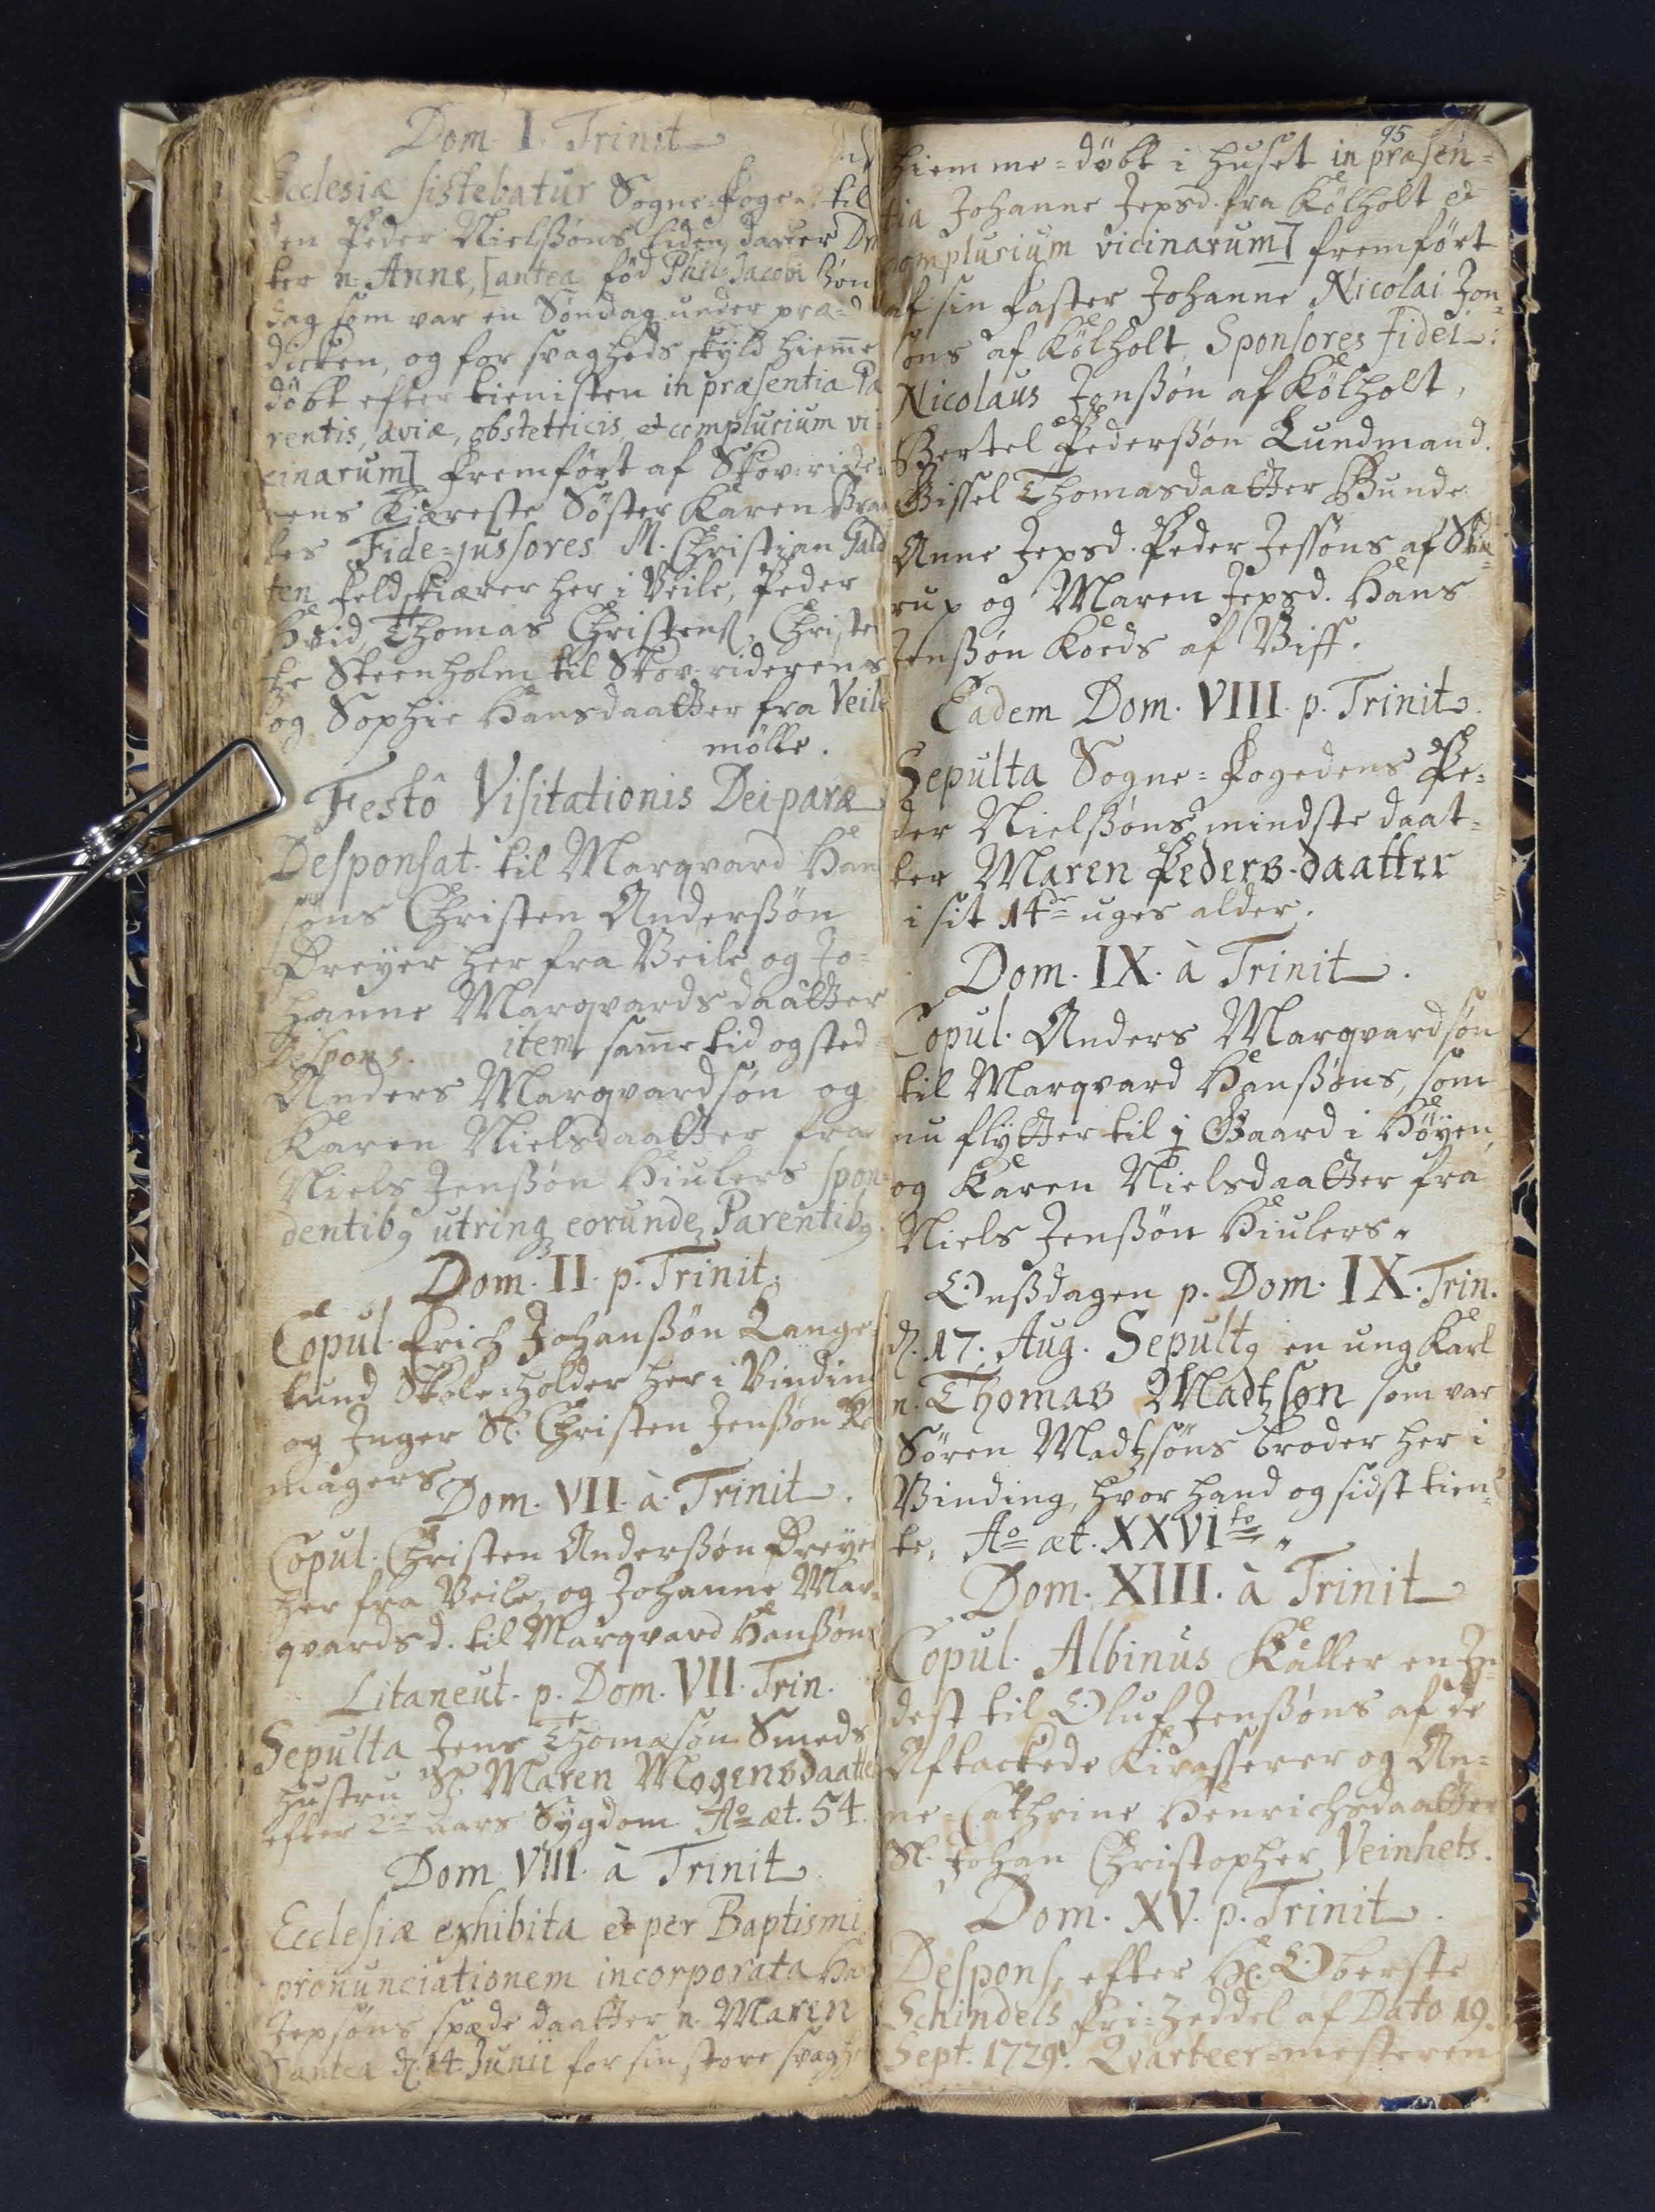Dom. I. Trinit
Ecclesiæ sistebatur Sogne=Foge 
en Peder Nielssøns liden daa 
ter n. Anna, [antea fød Philip Jacobi
dag som var en Søndag under præ¬
dicken, og for svagheds skyld hiemme
døbt efter tienisten in præsentia Pa
rentis, aviæ, obstetricis et complurium vi¬
cinarum] fremført af Skov=ride¬
ens Kiæreste Søster Karen Braa 
tes Fide=jussores M. Christian Gald
ten Feldskiærer her i Veile, Peder
Hvid, Thomas Christens, Christe 
ze Steenholm til Skov=riderns
og Sophie Hansdaatter fra Veile
Mølle.
Festo Visitationis Dei-paræ
Desponsat til Marqvard Han
søns Christen Anderssøn
Dreyer her fra Veile og Jo¬
hanne Marqvardsdaatter
Despons.
item samme tid og sted
Anders Marqvardsøn og
Karen Nielsdaatter fra
Niels Jenssøn Hiulers Spon¬
dentibq utringz eorundez Parentibq
Dom. II. p. Trinit
Copul. Erich Johanssøn Lange¬
lund Skole=holder her i Vindin 
og Inger Sl. Christen Jenssøn Rø 
magers.
Dom. VII. a. Trinit
Copul. Christen Anderssøn Dreye 
her fra Veile, og Johanne Mar¬
qvardsd. til Marqvard Hanssøns
Litaneut. p. Dom. VII. Trin
Sepulta Jens Thomæsøn Smeds 
hustru Sl. Maren Mogensdaatter
efter 2de Aars Sygdom Ao æt. 54.
Dom. VIII. a Trinit
Ecclesiæ exhibita et per Baptismi 
pronunciationem incorporata Ha 
Jepsøns spæde daatter n. Maren 
[ antea d. 14 Junii for sin store svaghe 
95
hiemme = døbt i huset in præsen¬
tia Johanne Jepsd. fra Kølholt et 
complurium vicinarum] fremført
af sin Faster Johanne Nicolai Jon¬
søns af Kølholt Sponsores fidei
Nicolaus Jonssøn af Kølholt
Bertel Pederssøn Lundmand.
Gissel Thomasdaatter Bunde
Anne Jepsd. Peder Jessøns af
rup og Maren Jepsd. Hans
Jenssøn Koeds af Viff
Eadem Dom. VIII. p. Trinit
Sepulta Sogne=Fogedens Pe¬
der Nielssøns mindste daat¬
ter Maren Peders-daatter
i sit 14de uges alder.
Dom. IX. a Trinit.
Copul. Anders Marqvardsøn
til Margvard Hanssøns, som
nu flytter til 1 Gaard i Høyen
og Karen Nielsdaatter fra
Niels Jenssøn Hiulers.
Onssdagen p. Dom. IX .Trin.
d. 17. Aug. Sepultq en ung Karl
n. Thomas Madtzsøn som var
Søren Madtzsøns broder her i
Vinding, hvor hand og sidst tien¬
te, Ao æt. XXVIto
Dom. XIII. a Trinit
Copul. Albinus Kaller en In¬
dest til Oluf Jenssøns af de
Aftackede Kirasserer og An¬
ne = Cathrine Hentichsdaatter
Sl. Johan Christropher Veinhets.
Dom. XV. p. Trinit
Despons efter Hl Oberste
Schindels fri=Zeddel af Dato 19.
Sept. 1729 Qvarteer=mesteren
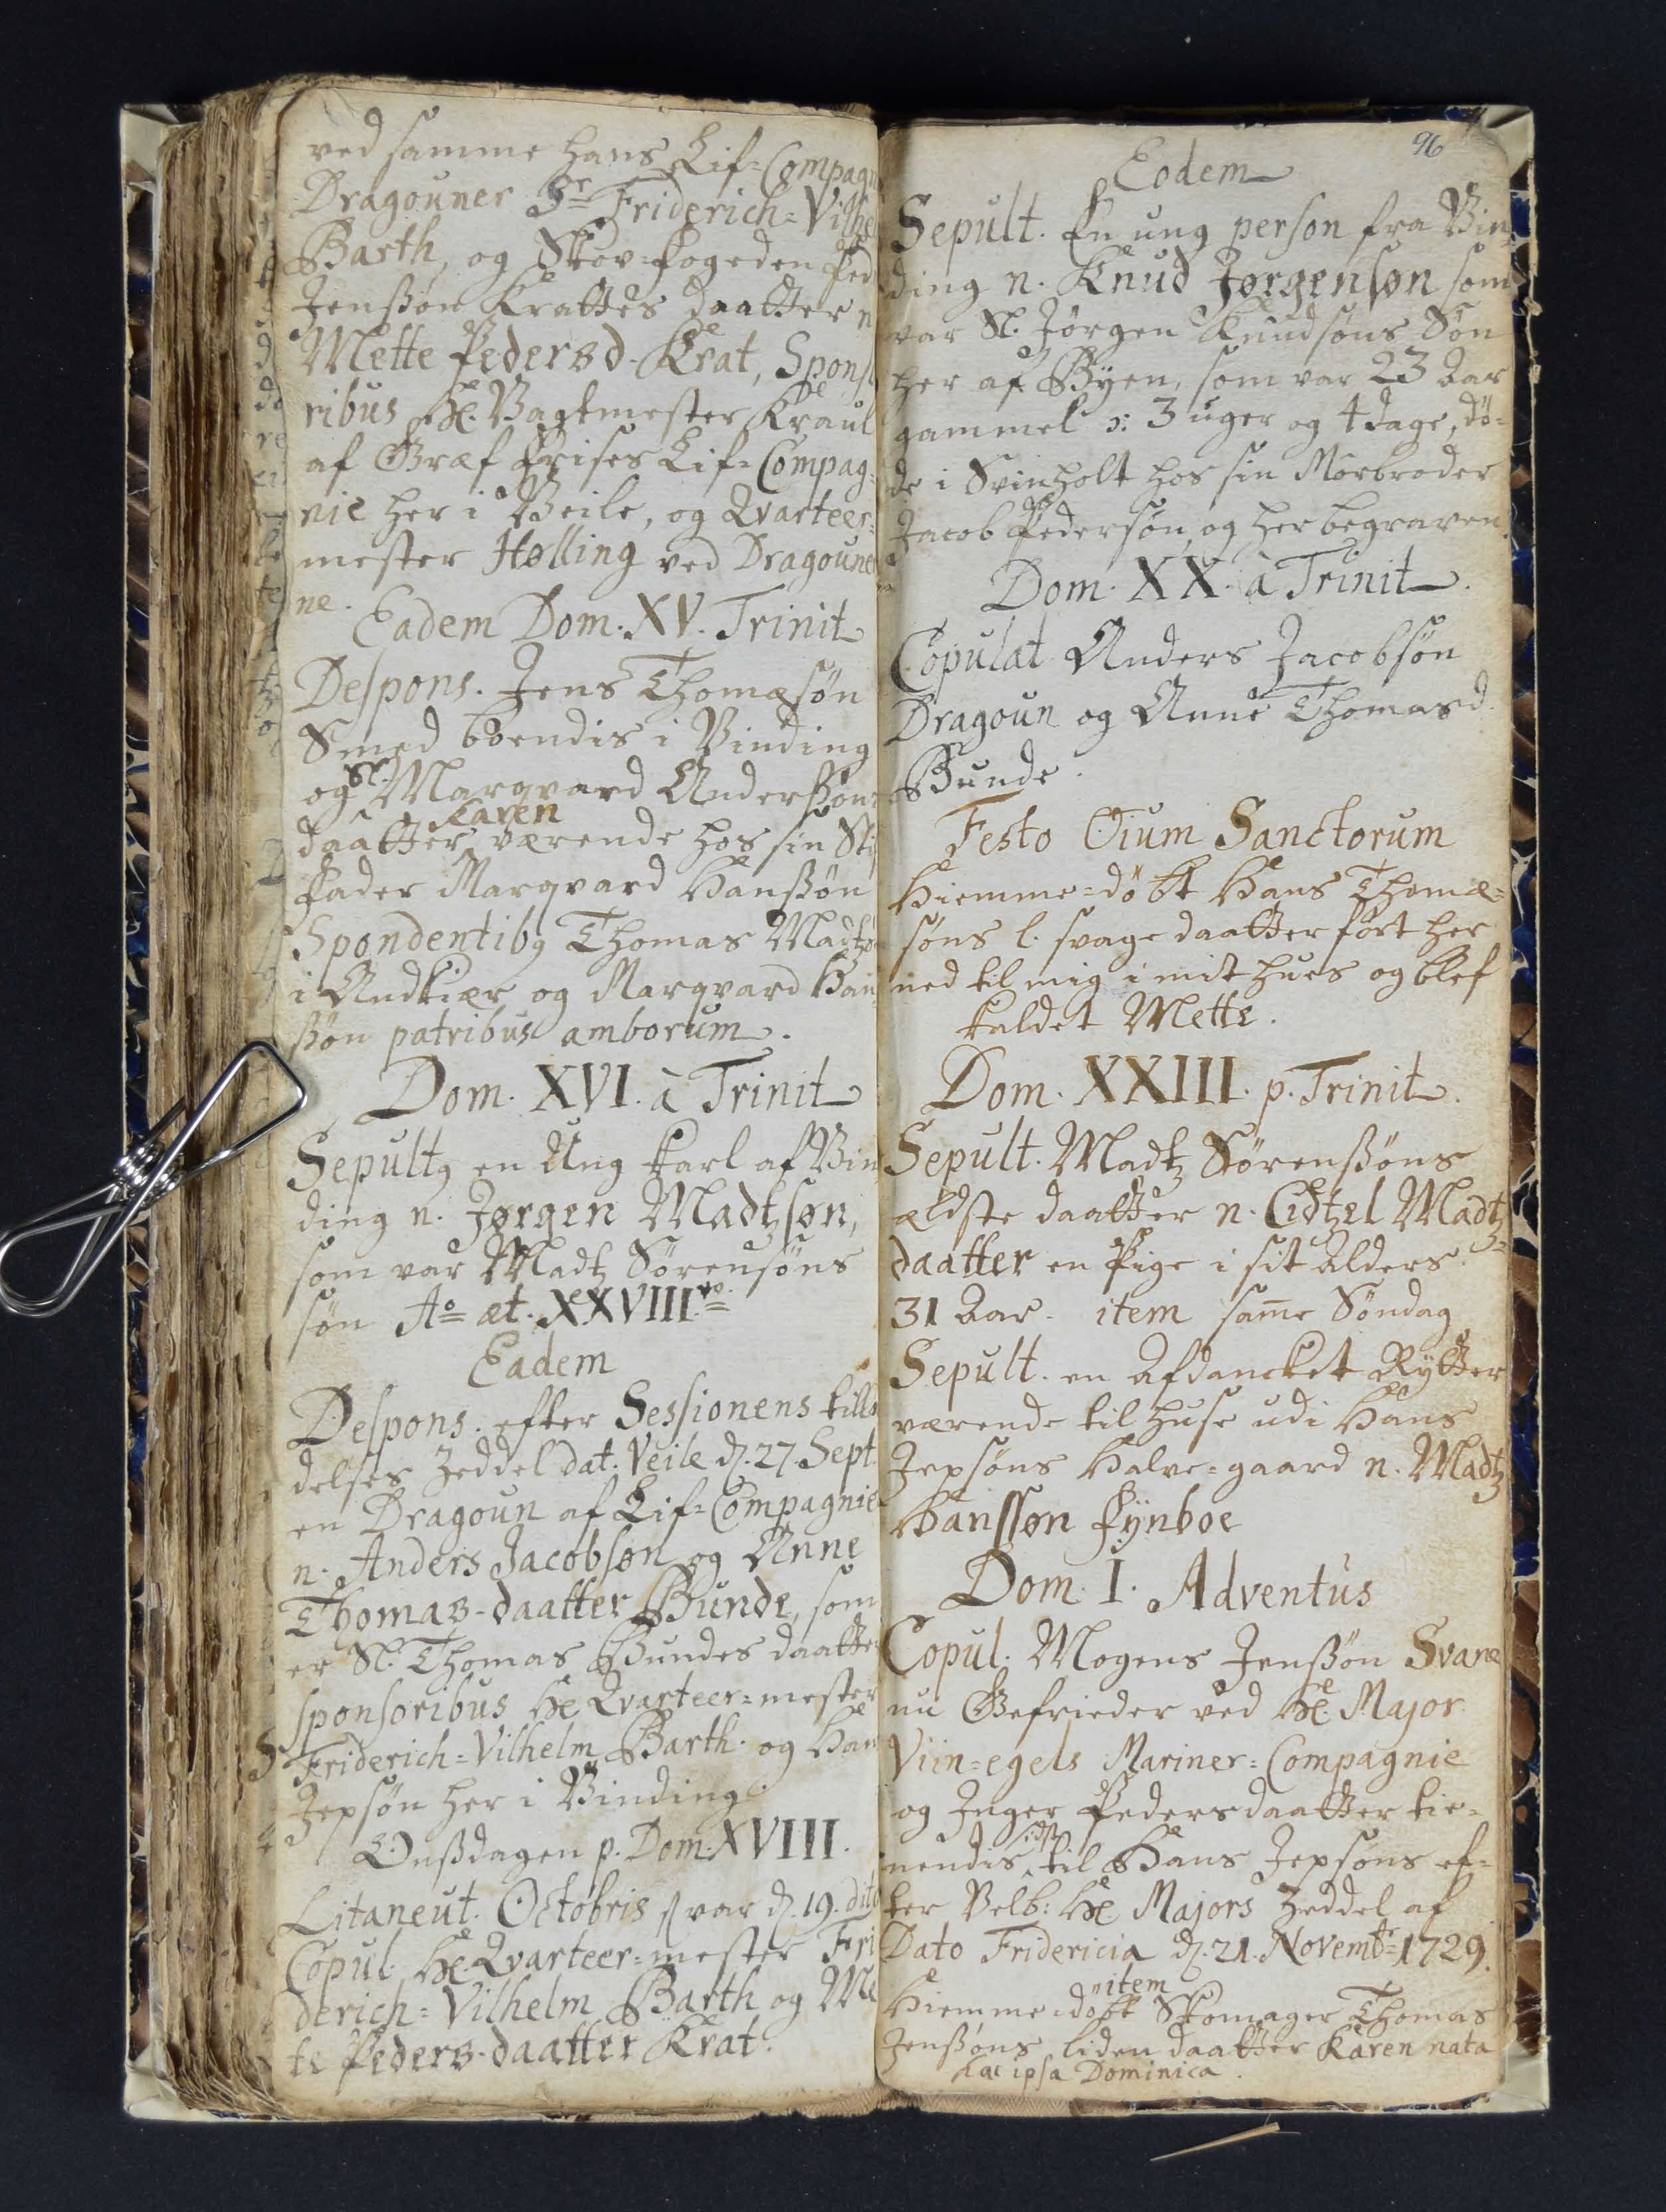ved samme hans Lif=Compagn 
Dragouner Sr Friderich = Vilhe 
Barth og Skovfogeden Ped 
Jenssøn Krattes daatter n
Mette Pedersda - Krat. Spons 
ribus Hl Vagtmester Kraul
af Græf Frises Lif=Compag¬
nie her i Veile, og Qvarteer¬
mester Hølling ved Dragoune 
ne.
Eadem Dom. XV. Trinit
Despons. Jens Thomæsøn
Smed boendis i Vinding
Sl.
og Marqvard Anderssøns
Karen
daatter værende hos sin Stif
Fader Marqvard Hanssøn
Spondentibq Thomas Madtzøn 
i Andkiær og Marqvard Han¬
ssøn patribus amborum.
Dom. XVI. a Trinit
Sepultq en Ung Karl af Vin
ding n. Jørgen Madtzsøn 
som var Madtz Sørensøns
søn Ao æt. XXVIII##o
Eadem
Despons. efter Sessionens tilla
delse Zeddel dat. Veile d. 27 Sept. 
en Dragoun af Lif=Compagnie 
n. Anders Jacobsøn og Anne
Thomas-daatter Bunde, som
er Sl. Thomas Bundes daatter 
Sponsoribus Hl Qvarteer=mester
Friderich=Vilhelm Barth, og Han 
Jepsøn her i Vinding
Onssdagen p. Dom. XVIII.
Litaneut. Octobris ## var d. 19 dito
Copul. Hl Qvarteer=mester Fri
derich=Vilhelm Barth og Me  
te Peders-daatter Krat.
96
Eodem
Sepult. En ung person fra Vin¬
ding n. Knud Jørgensøn som 
var Sl. Jørgen Knudsøns Søn
her af Byen, som var 23 Aar
gammel ↄ: 3 uger og 4 dage, dø¬
de i Svinholt hos sin Morbroder
Jacob Pedersøn, og her begraven
Dom. XX. a Trinit
Copulat Anders Jacobsøn
Dragoun og Anne Thomasd
Bunde
Festo Oium Sanctorum
Hiemme=døbt Hans Thomæ¬
søns l. svage daatter, ført her 
ned til mig i mit Hues og blef 
kaldet Mette.
Dom. XXIII. p. Trinit.
Sepult. Madtz Sørenssøns
ældste daatter n. Cidtzel Madtz¬
daatter en Pige i sit Alders.
31 Aar. item samme Søndag
Sepult. en Afdancket Rytter
værende til huse udi Hans
Jepsøns Halve=gaard n. Madtz 
Hanssøn Fynboe
Dom. I. Adventus
Copul. Mogens Jenssøn Svane
nu Gefrieder ved Hl Major
Viin=egels Mariner=Compagnie 
og Inger Pedersdaatter tie¬
sidst
nendis sidst til Hans Jepsøns ef¬
ter Velb: Hl Majors Zeddel af
Dato Fridericia d. 21. Novemb = 1729
item
Hiemme=døbt Skomager Thomas
Jenssøns liden daatter Karen nata
ipsa Dominica